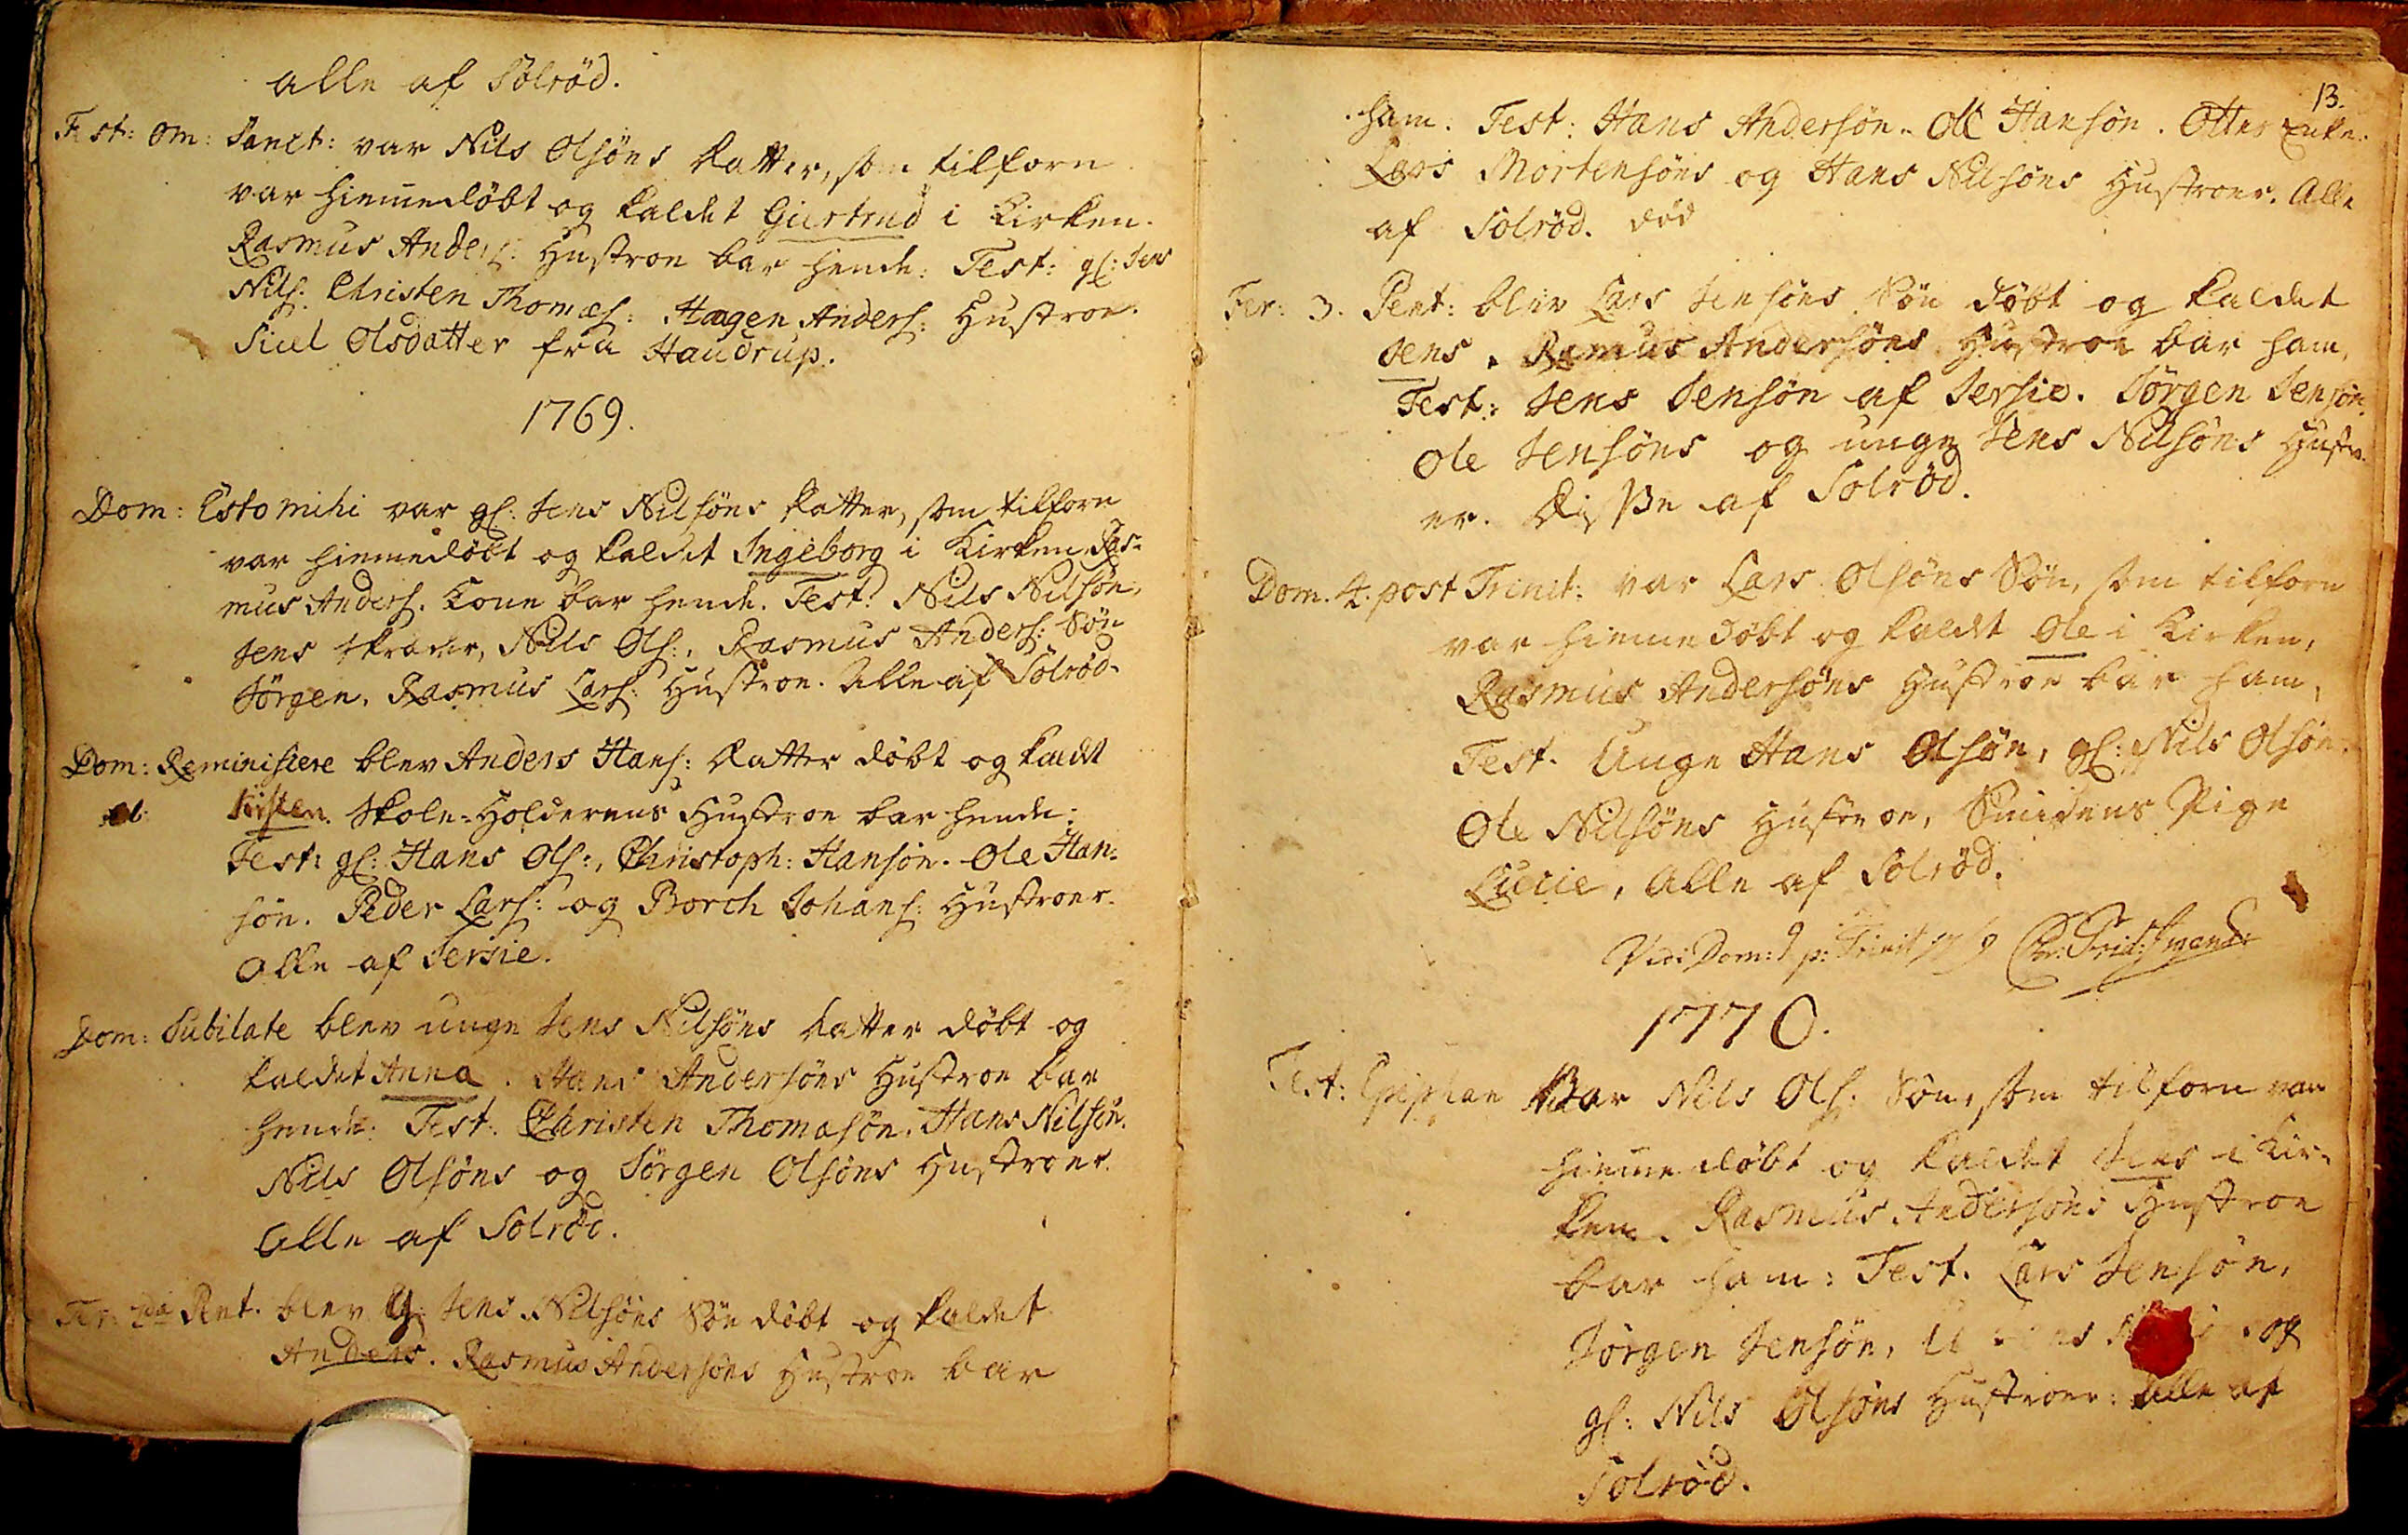Alle af Solrød.
Fest: Om: Sanct: var Nils Olsøns Datter, som tilforn
var hiemedøbt og kaldet Giertrud i Kirken.
Rasmus Anders: Hustroe bar hende: Test gl: Jens
Nils: Christen Thomæs: Haagen Anders: Hustroe.
Sicel Olsdatter fra Haudrup.
1769.
Dom: Esto mihi var gl: Jens Nilsøns Datter, som tilforn
var hiemmedøbt og kaldet Ingeborg i Kirken Ras¬
mus Anders. Kone bar hende. Test: Nils Nilsøn,
Jens skræder, Nils Ols: Rasmus Anders: Søn
Jørgen. Rasmus Lars: Hustroe. Alle af Solrød.
Dom: Reminiscere blev Anders Hans: Datter døbt og kaldet
Kirsten. Skole=Holderens Hustroe bar hende:
Test: gl: Hans Ols: Christoph: Hansøn. Ole Han
søn. Peder Lars: og Borch Johans: Hustroer.
Alle af Jersie.
Dom: Jubilate blev unge Jens Nilsøns Datter døbt og
kaldet Anna. Hans Andersøns Hustroe bar
hende: Test: Christen Thomæsøn, Hans Nilsøn,
Nils Olsøns og Jørgen Olsøns Hustroer.
Alle af Solrød
Fer. 2da Pent: blev gl: Jens Nilsøns Søn døbt og kaldet
Anders. Rasmus Andersøns Hustroe bar
13.
ham: Test: Hans Andersøn. Ole Hansøn. Ottes Enke
Lars Mortensøns og Hans Nilsøns Hustroer. Alle
af Solrød. død
Fer: 3. Pent: blev Lars Jensøns Søn døbt og kaldet
Jens. Rasmus Andersøns Hustroe bar ham,
Test: Jens Jensøn af Jersie. Jørgen Jensøn.
Ole Jensøns og unge Jens Nilsøns Hustro
er: Disse af Solrød.
Dom. 4. post Trinit: var Lars Olsøns Søn, som tilforn
var hiemmedøbt og kaldet Ole i Kirken,
Rasmus Andersøns Hustroe bar ham,
Test. Unge Hans Olsøn, gl: Nils Olsøn.
Ole Nilsøns Hustroe, Smidens Pige
Lucie, Alle af Solrød.
Vidi Dom: 9 p:Trinit 1769 Chr:Frid:Jrgens.
1770.
Fest: Epiphan var Nils Ols: Søn, som tilforn var
hiemedøbt og kaldet Jens i Kir¬
ken. Rasmus Andersøns Hustroe
bar ham: Test: Lars Jensøn,
Jørgen Jensøn, Unge Jens ## og
gl: Nils Olsøns Hustroer: Alle af
Solrød. 
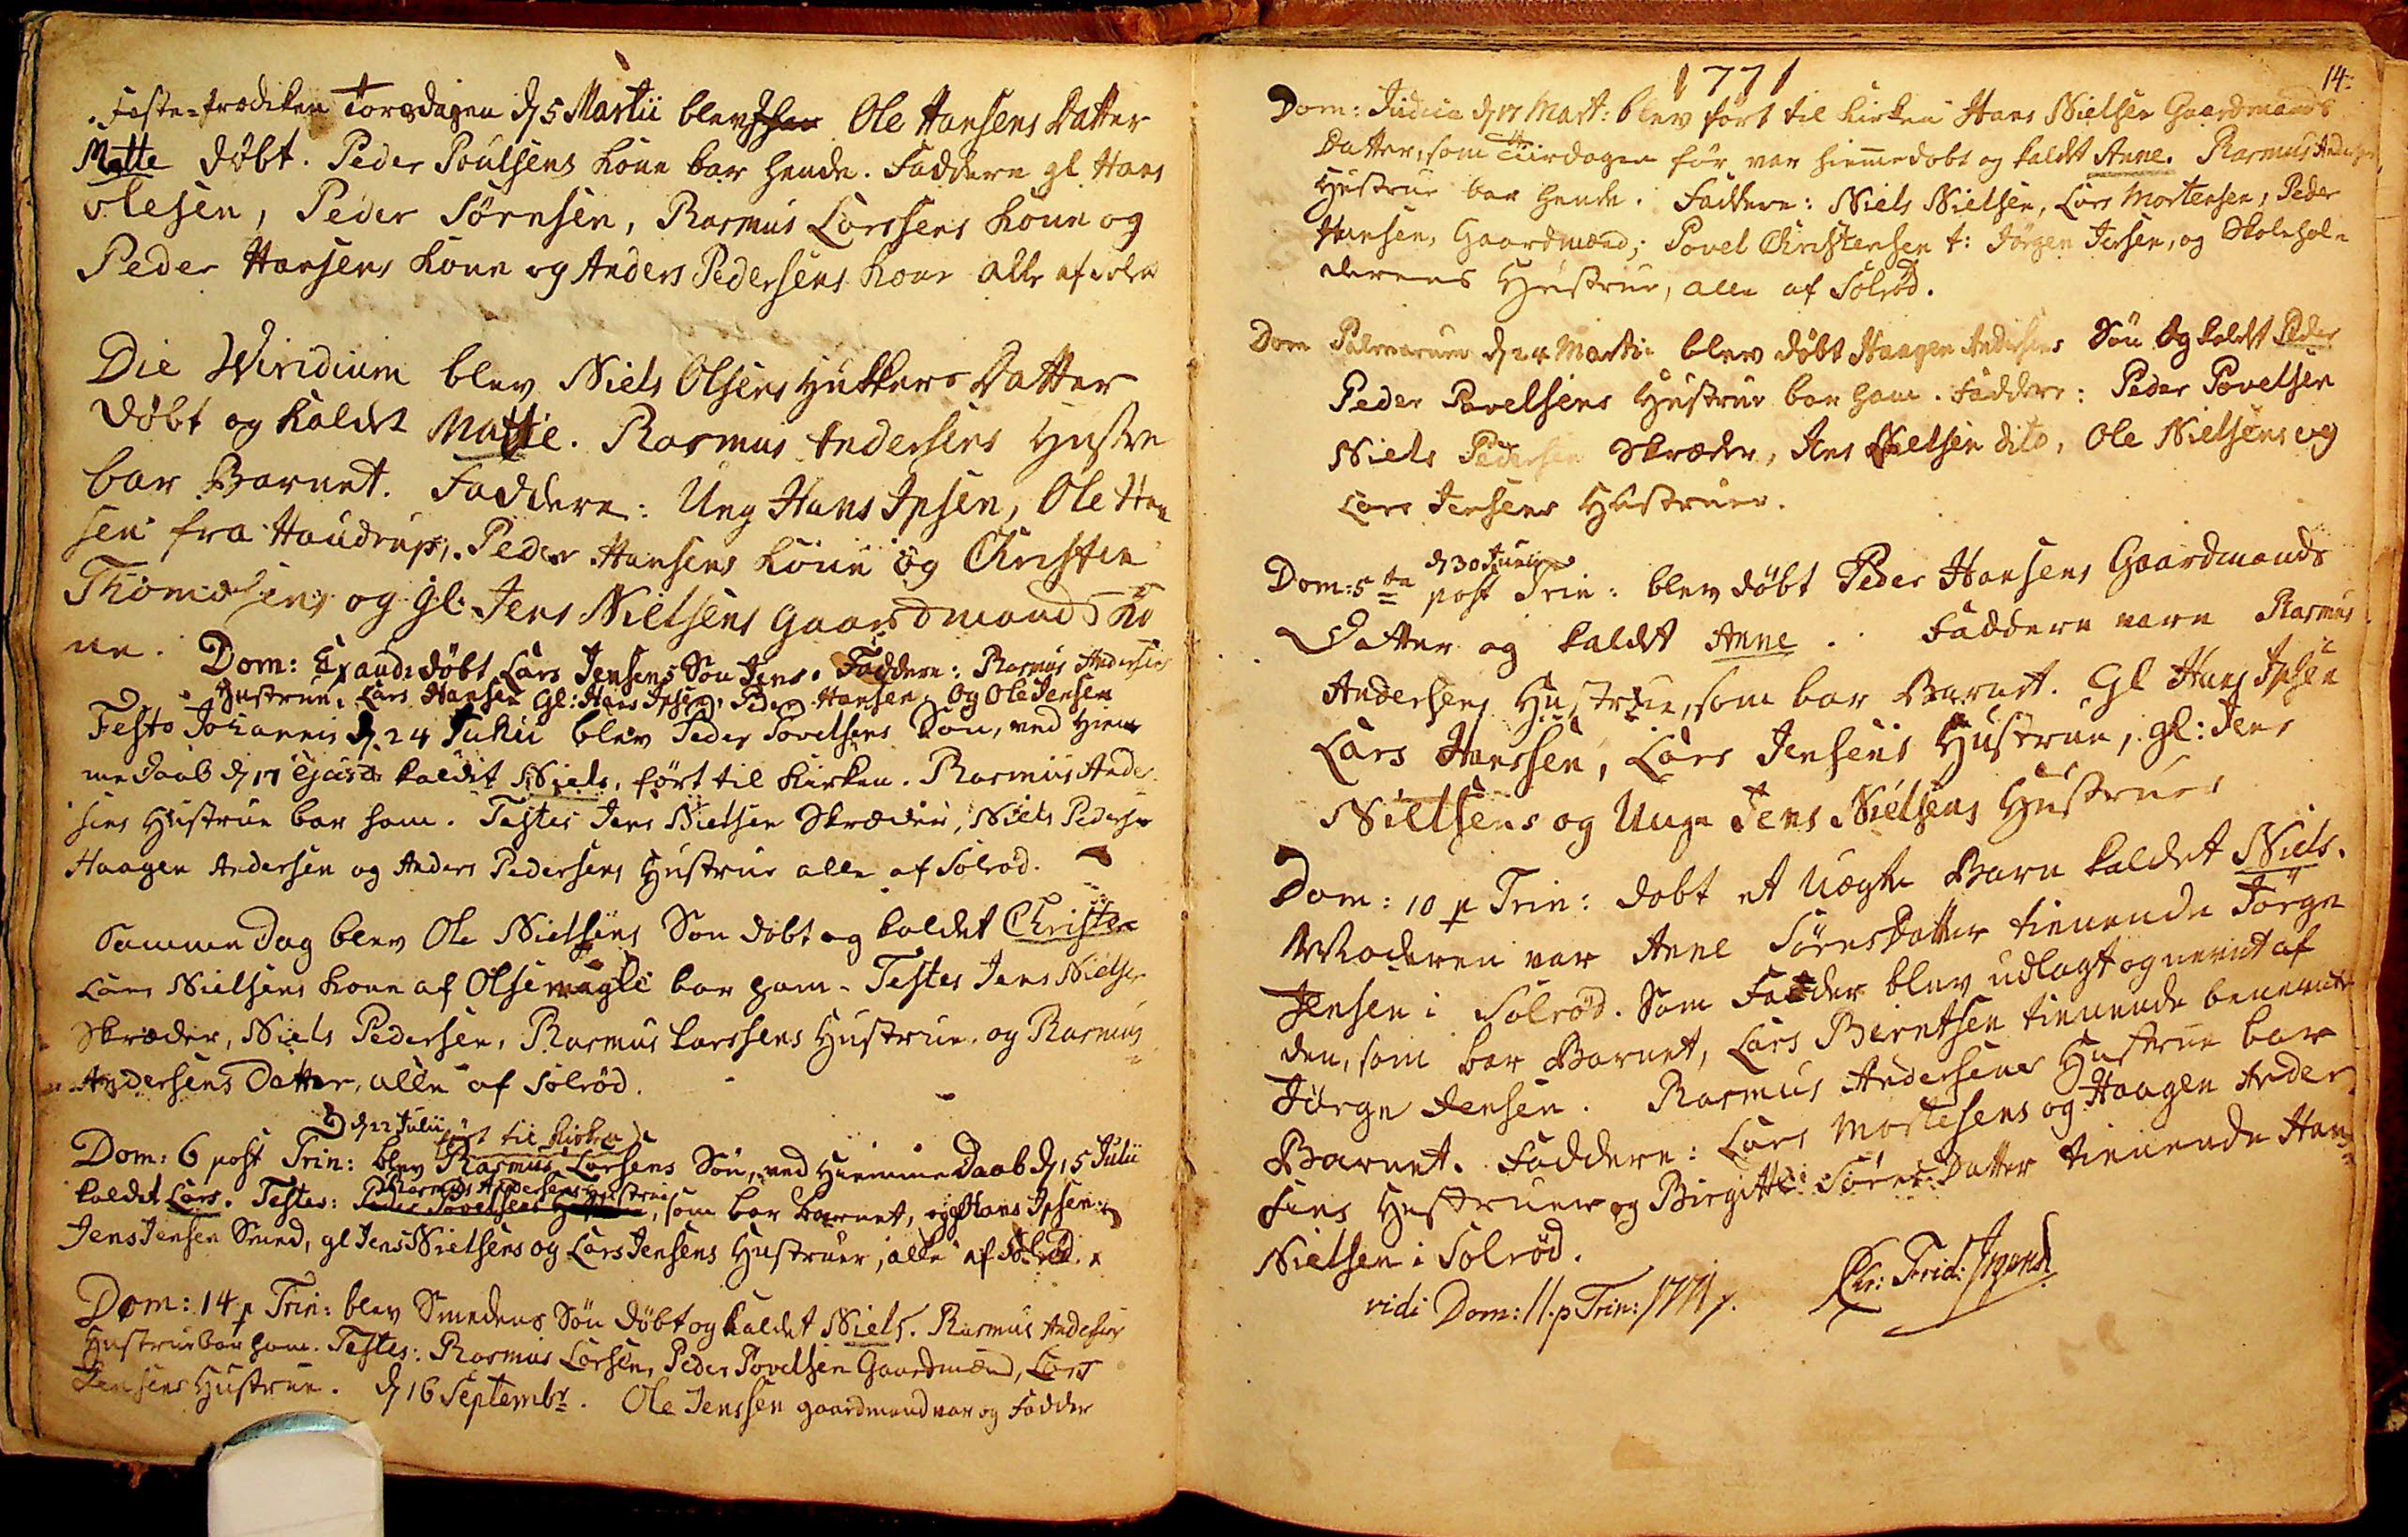Faste=prædiken Torsdagen d 5 Martii blev Johan Ole Hansens Datter
Mette døbt. Peder Poulsens Kone bar hende. Faddere gl Hans
Olsen, Peder Sørnsen, Rasmus Larssens Kone og
Peder Hansens Kone og Anders Pedersens Kone alle af Solrød
Die Wiridium blev Niels Olsens Huggers Datter
døbt og kaldt Mette. Rasmus Andersens Hustru
bar Barnet. Faddere: Ung Hans Ipsen, Ole Han¬
sen fra Haudrup. Peder Hansens Kone og Christen
Thomæsens og gl. Jens Nielsens Gaardmands Ko
ne. Dom: Exaudi døbt Lars Jensens Søn Jens. Faddere: Rasmus Andersens
Hustrue: Lars Hansen gl: Hans Ipsen, Peder Hansen, og Ole Jensen
Festo Johannes d. 24 Junii blev Peder Povelsens Søn, ved hiem
me Daab d 17 Ejusd: kaldet Niels, ført til Kirken. Rasmus Ander
sens Hustrue bar ham. Testes Jens Nielsen Skræder, Niels Pedersen
Haagen Andersen og Anders Pedersens Hustrue alle af Solrød.
Samme Dag blev Ole Nielsens Søn døbt og kaldet Christen
Lars Nielsens Kone af Ølsemagle bar ham. Testes Jens Nielsen
Skræder, Niels Pedersen, Rasmus Larsens Hustrue, og Rasmus
Andersens Datter, alle af Solrød
d 22 Julii ført til Kirken
Dom: 6 post Trin: blev Rasmus Larsens Søn, ved HiemmeDaab d 15 Julii
Rasmus Andersens Hustrue
kaldet Lars. Testes Peder Povelsens Hustrue, som bar Barnet, og Hans Ipsen,
Jens Jensen Smed, gl Jens Nielsens og Lars Jensens Hustruer, alle af Solrød.
Dom: 14 p Trin: blev Smedens Søn døbt og kaldet Niels. Rasmus Andersens
Hustrue bar ham. Testes: Rasmus Larsen, Peder Povelsen Gaardmænd, Lars
Jensens Hustrue. d 16 Septembr. Ole Jensen Gaardmand var og Fadder
14.
1771
Dom: Judica d 17 Mart: blev ført til Kirken Hans Nielsen Gaardmands
Datter, som Tiirsdagen før var hiemedøbt og kaldt Anne. Rasmus Andersens
Hustrue bar hende. Faddere. Niels Nielsen, Lars Mortensen, Peder
Hansen, Gaardmænd; Povel Christensen t: Jørgen Jensen, og Skolehol¬
derens Hustrue, Alle af Solrød.
Dom Palmarum d 24 Martii blev døbt Haagen Andersens Søn og kaldt Peder
Peder Povelsens Hustrue bar ham. Faddere: Peder Povelsen
Niels Pedersen Skræder, Jens Nielsen dito, Ole Nielsens og
Lars Jensens Hustruer.
d 30 Junii
Dom: 5ta post Trin: blev døbt Peder Hansens Gaardmands
Datter og kaldt Anne. Faddere vare Rasmus
Andersens Hustrue, som bar Barnet. Gl Hans Ipsen
Lars Hanssen, Lars Jensen Hustrue, gl: Jens
Nielsens og Unge Jens Nielsens Hustruer.
Dom: 10 p Trin: døbt et Uægte Barn kaldet Niels.
Moderen var Anne Sørnsdatter tienende Jørgn
Jensen i Solrød. Som Fadder blev udlagt og nevnt af
den, som bar Barnet, Lars Berntsen tienende benevnte
Jørgn Jensen. Rasmus Andersens Hustrue bar
Barnet. Faddere: Lars Mortesens og Haagen Ander
sens Hustruer og Birgitte SørnsDatter tienende Hans
Nielsen i Solrød.
vidi Dom: 11.p Trin. 1771.
Chr: Frid: Irgens.